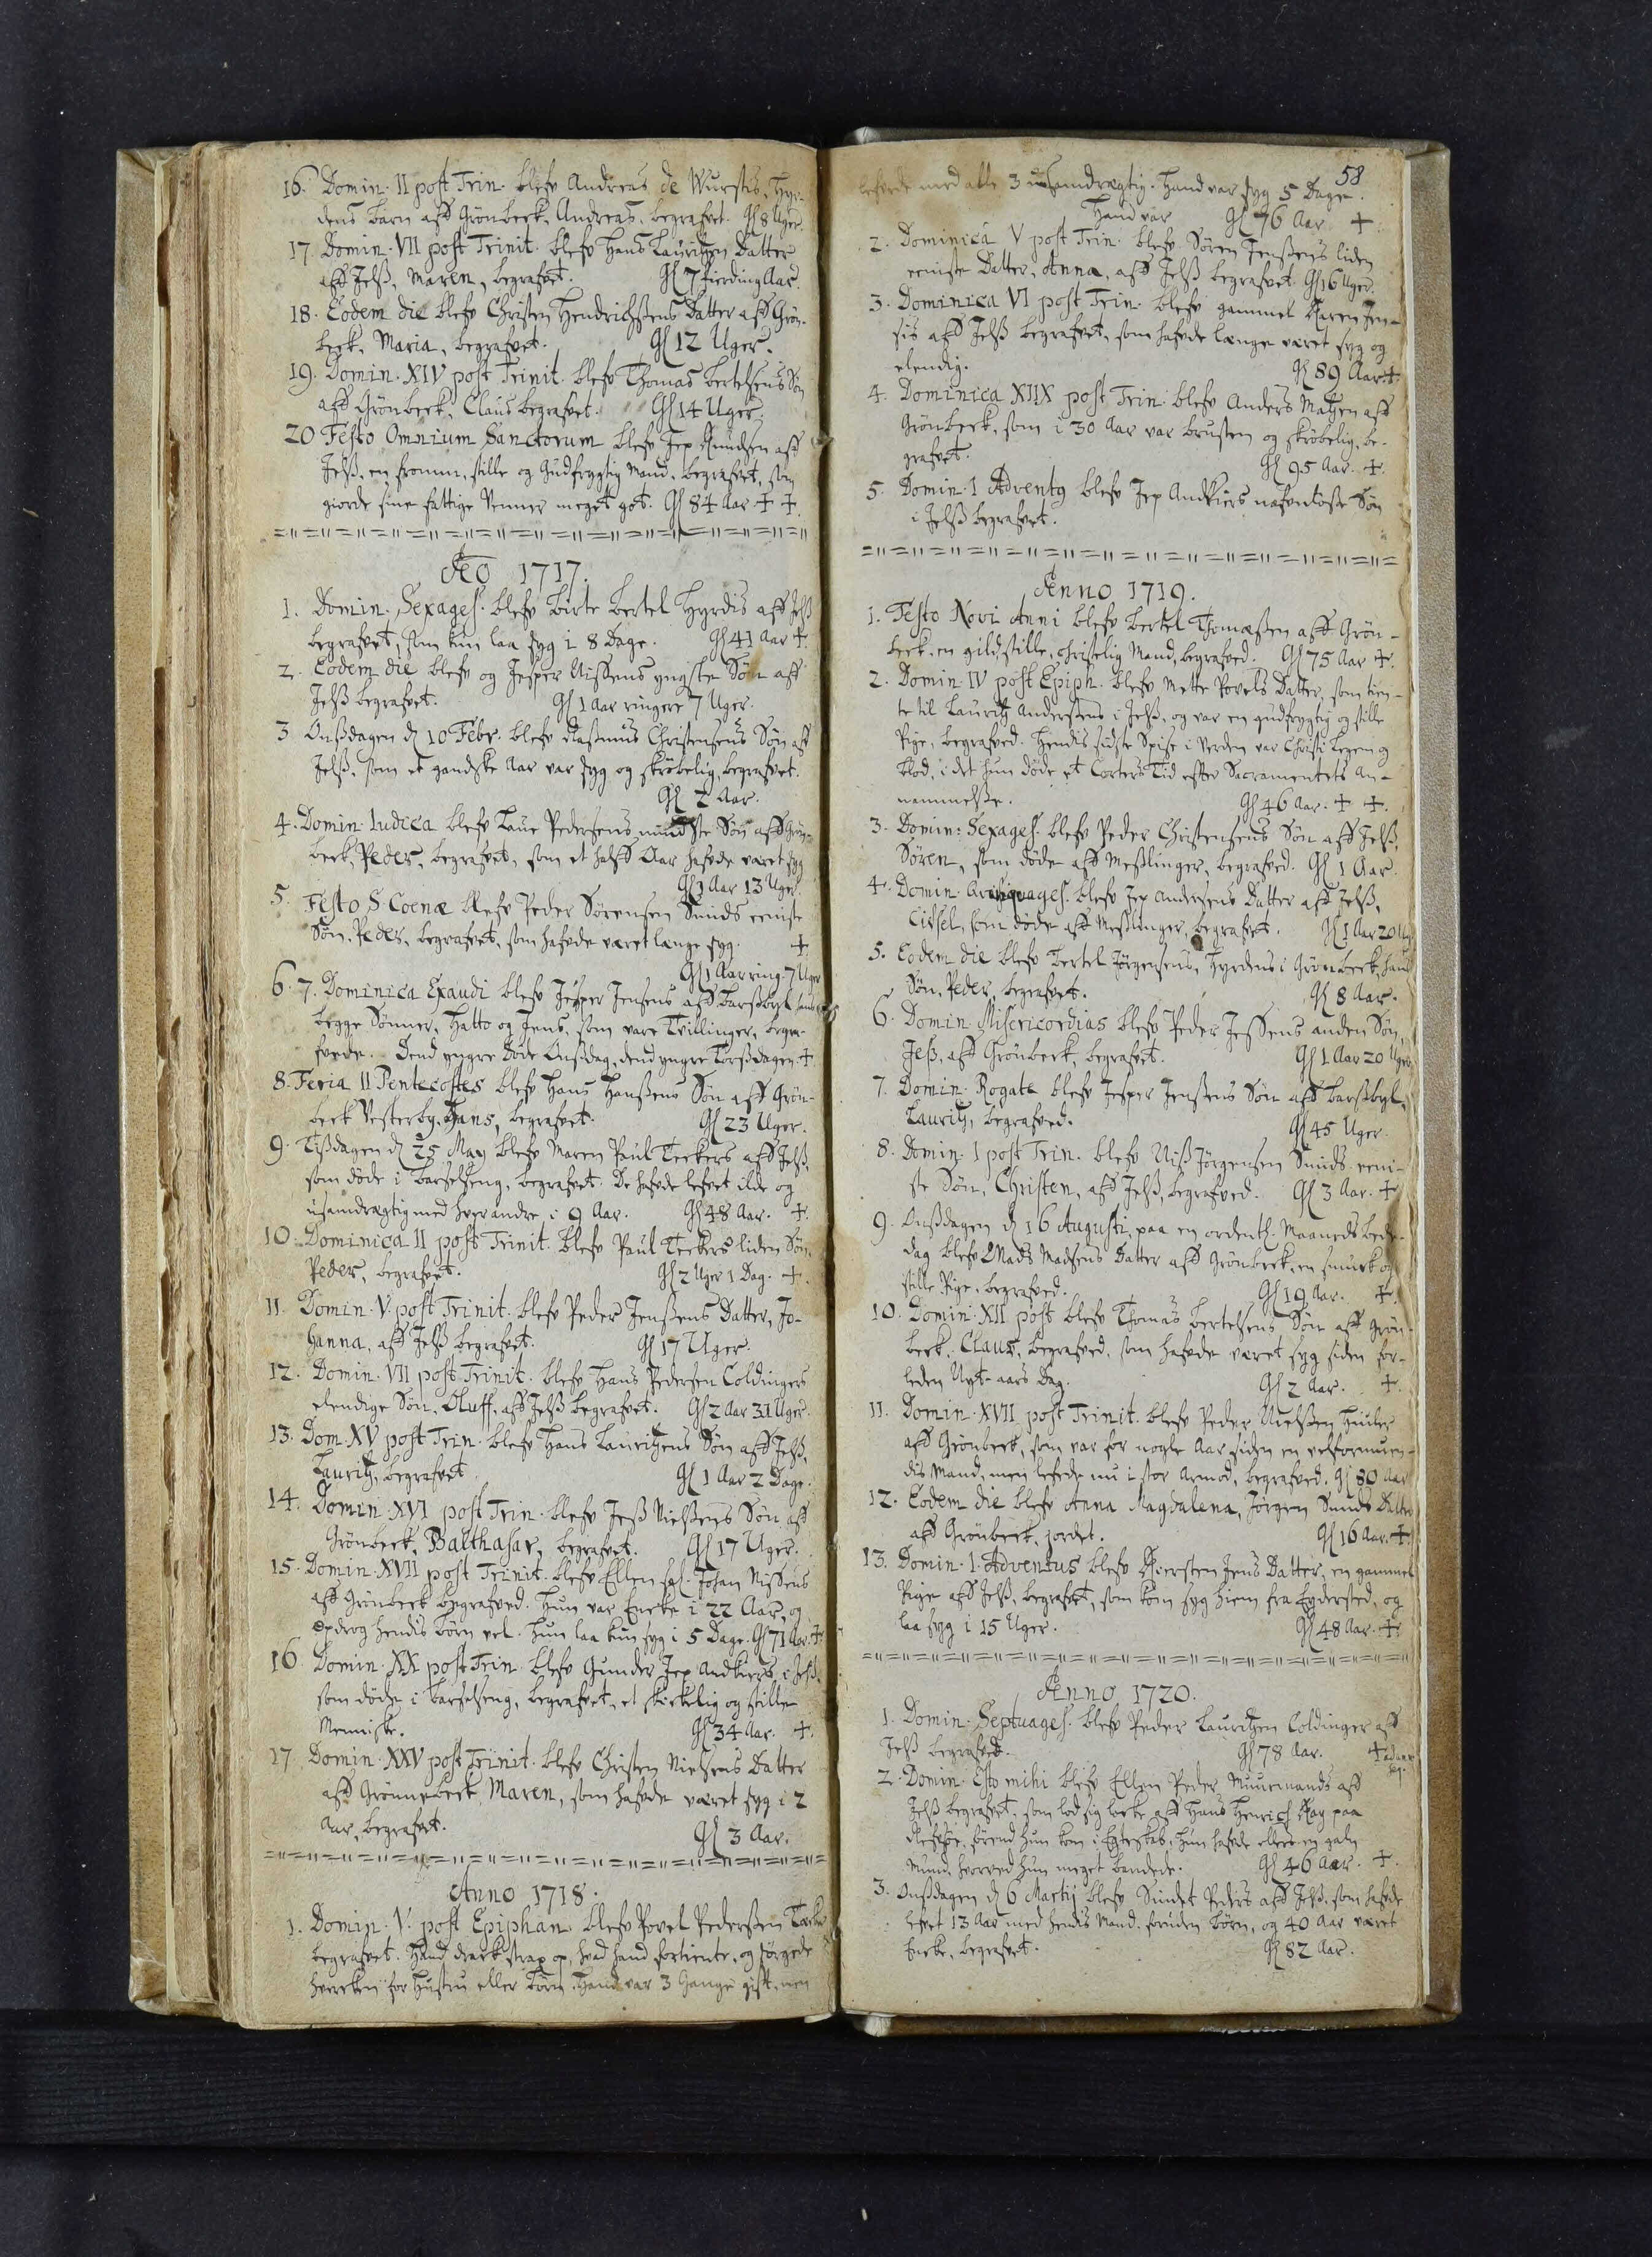16. Domin. II post Trin blefv Andreas de Wurstis, Hyr¬
dens barn aff Grønbeck, Andreas, begrafvet. Gl 8 Uger.
17. Domin. VII post Trinit. blefv Hans Lauritzen Datter
aff Jelss, Maren, begrafvet.
Gl 7 Fierding Aar.
18. Eodem die blefv Christen Hendrichssens Datter aff Grøn¬
beck, Maria, begrafvet.
Gl 12 Uger.
19. Domin. XIV post Trinit blefv Thomas Bertelsens Søn
aff Grønbeck, Claus begrafvet. Gl 14 Uger
20. Festo Omnium Sanctorum blefv Jep Knudsen aff
Jelss, en fromm, stille og Gudfrygtig Mand, begrafvet, som
giorde sine fattige Venner meget got. Gl 84 Aar
Ao 1717.
1. Domin. Sexages. blefv Birte Bertel Hyrdis aff Jelss
begrafvet, som kun laa syg i 8 Dage. Gl 41 Aar †.
2. Eodem die blefv og Jesper Nissens yngste Søn aff
Jelss begrafvet.
Gl 1 Aar ringere 7 Uger.
3. Onssdagen d 10 Febr. blefv Rassmus Christensens Søn aff
Jelss. som et gandske Aar var syg og skrøbelig, begrafvet.
Gl 2 aar.
4. Domin. Iudica blefv Laue Pedersens mindste Søn aff Grøn¬
beck, Peder, begrafvet, som et halff Aar hafvde varet syg
Gl 1 Aar 13 Uger.
5. Festo S. Coenæ blefv Peder Sørensen Smids eeniste
Søn, Peder, begrafvet, som hafvde varet længe syg
Gl 1 Aar ring. 7 Uger
6. 7. Dominica Exaudi blefv Jesper Jensens aff Barsbyl
begge Sønner, Hatto og Jens, som vare Tvillinger, begra¬
fvede. Dend yngre døde Onssdag, den yngre Torssdagen
8. Feria II Pentecostes blefv Hans Hanssens Søn aff Grøn¬
beck Vesterby, Hans, begrafvet.
Gl 23 Uger.
9. Tissdagen d 25 May blefv Maren Paul Teckers aff Jelss,
som døde i Barselseng, begrafvet. De hafvde lefvet ilde og
usamdrægtig med hver andre i 9 Aar. Gl 48 Aar.
10. Dominica II post Trinit. blefv Paul Teckers liden Søn
Peder, begrafvet
Gl 2 Uger 1 Dag. †.
11. Domin. V post Trinit. blefv Peder Jenssens Datter, Jo¬
hanna, aff Jelss begrafvet.
Gl 17 Uger
12. Domin. VII post Trinit. blefv Hans Pedersen Coldingers
elendige Søn, Oluff, aff Jelss begrafvet. Gl 2 Aar 31 Uger
13. Dom. XV post Trin. blef Hans Lauritzens Søn aff Jelss,
Lauritz, begrafvet.
Gl 1 Aar 2 Dage.
14. Domin. XVI post Trin. blefv Jess Nielssens Søn aff
Grønbeck, Balthasar, begrafvet. Gl 17 Uger.
15. Domin. XVII post Trinit. blefv Ellen sal. Johan Nissens
aff Grønbeck begrafved. Hun var Encke i 22 Aar, og
opdrog hendis Børn vel. Hun laa kun syg i 5 Dage. Gl 71 Aar. †.
16. Domin. XX post Trin. blefv Gunder Jep Andkiers i Jelss,
som døde i barselseng, begrafvet, et skickelig og stille
Menniske.
Gl 34 Aar.
17. Domin. XXV post Trinit. blefv Christen Nielsens Datter
aff Grønnebeck, Maren, som hafvde været syg i 2
Aar, begrafvet.
Gl 3 Aar.
Anno 1718.
1. Domin. V post Epiphan. blefv Povel Pederssen Tæcker
begrafet. Hand drack strax op, hvad hand fortiente, og sørgede
hvercken for Hustru eller Børn. Hand var 3 gange gift, men
58
lefvede med alle 3 usamdrægtig. Hand var syg 5 Dage.
Hand var Gl 76 Aar.
2. Dominica V post Trin. blefv Søren Jenssens liden
eeniste Datter, Anna, aff Jelss begrafvet. Gl 16 Uger.
3. Dominica VI post Trin. blefv gammel Karen Jen¬
sis aff Jelss begrafvet, som hafvde længe været syg og
elendig.
Gl 89 Aar. †.
4. Dominica XIIX post Trin. blefv Anders Matzen aff
Grønbeck, som i 30 Aar var brusten og skrøbelig, be¬
grafvet.
Gl 95 Aar. †.
5. Domin. I Adventq blefv Jep Andkiers nafvnløse Søn
i Jelss begrafvet.
Anno 1719.
1. Festo Novi Anni blefv Bertel Thomasen aff Grøn¬
beck, en gild, stille, christelig Mand, begrafved. Gl 75 Aar
2. Domin. IV post Epiph. blefv Mette Povels Datter, som tien¬
te til Lauritz Andersen i Jelss, og var en gudfrygtig og stille
Pige, begrafved. Hendis sidste Spise i Verden var Christi Legem og
Blod, i det hun døde et Corters Tid efter Sacramentets An¬
nammelsse.
Gl 46 Aar.
3. Domin. Sexages. blefv Peder Christensens Søn aff Jelss,
Søren som døde aff Messlinger, begrafved. Gl 1 Aar.
4. Domin. Qvingvages. blefv Jep Andersens Datter aff Jelss,
Cidsel, som døde aff Meslsinger, begrafvet. Gl 1 Aar 20 Ug
5. Eodem die blefv Bertel Jørgensens, Hyrdens i Grønbeck, hans
Søn, Peder, begrafvet.
Gl 8 Aar.
6. Domin. Misericordias blefv Peder Jessens anden Søn,
Jess aff Grønbeck, begrafvet.
Gl 1 Aar 20 Uger
7. Domin. Rogate blefv Jesper Jenssens Søn aff Barssbyl,
Lauritz, begrafved.
Gl 45 Uger
8. Domin. I post Trin. blefv Niss Jørgensen Smids eeni¬
ste Søn, Christen, aff Jelss, begrafved. Gl 3 Aar.
9. Onssdagen d 16 Augusti, paa en ordentl. Maaneds bede¬
dag blefv Mads Madsens Datter aff Grønbeck, en smuck og
stille Pige, begrafved.
Gl 19 Aar.
10. Domin. XII post blefv Thomas Bertelsens Søn aff Grøn¬
beck, Claus, begrafved, som hafvde været syg siden for¬
leden Nyt-aars Dag.
Gl 2 Aar.
11. Domin. XVII post Trinit. blefv Peder Nielssen Hiuler
aff Grønbeck, som var for nogle Aar siden en velformuen¬
dis Mand, men lefede nu i stor Armod, begrafved. Gl 80 Aar
12. Eodem die blefv Anna Magdalena, Jørgen Smids Datter
aff Grønbeck, jordet.
Gl 16 Aar.
13. Domin. I Adventus blefv Kiersten Jens Datter, en gammel
Pige aff Jelss, begrafvet, som kom syg hiem fra Eydersted, og
laa syg i 15 Uger.
Gl 48 Aar. †
Anno 1720
1. Domin. Septuages. blefv Peder Lauritzen Coldinger aff
Jelss Begrafved.
Gl 78 Aar.
ad##
he##
2. Domin. Esto mihi blefv Ellen Peder Muurmands aff
Jelss begrafvet, som lod sig locke aff Hans Henrich Kay paa
Refsøe, førend hun kom i Egteskab, Hun hafvde ellers en
Mund. Hvorved hun meget bandede. Gl 46 Aar.
3. Onssdagen d 6 Martij blefv Sindet Peders aff Jelss, som hafvde
lefvet 13 Aar med hendis Mand, foruden Børn, og 40 Aar været
Encke, begrafvet.
Gl 82 Aar.